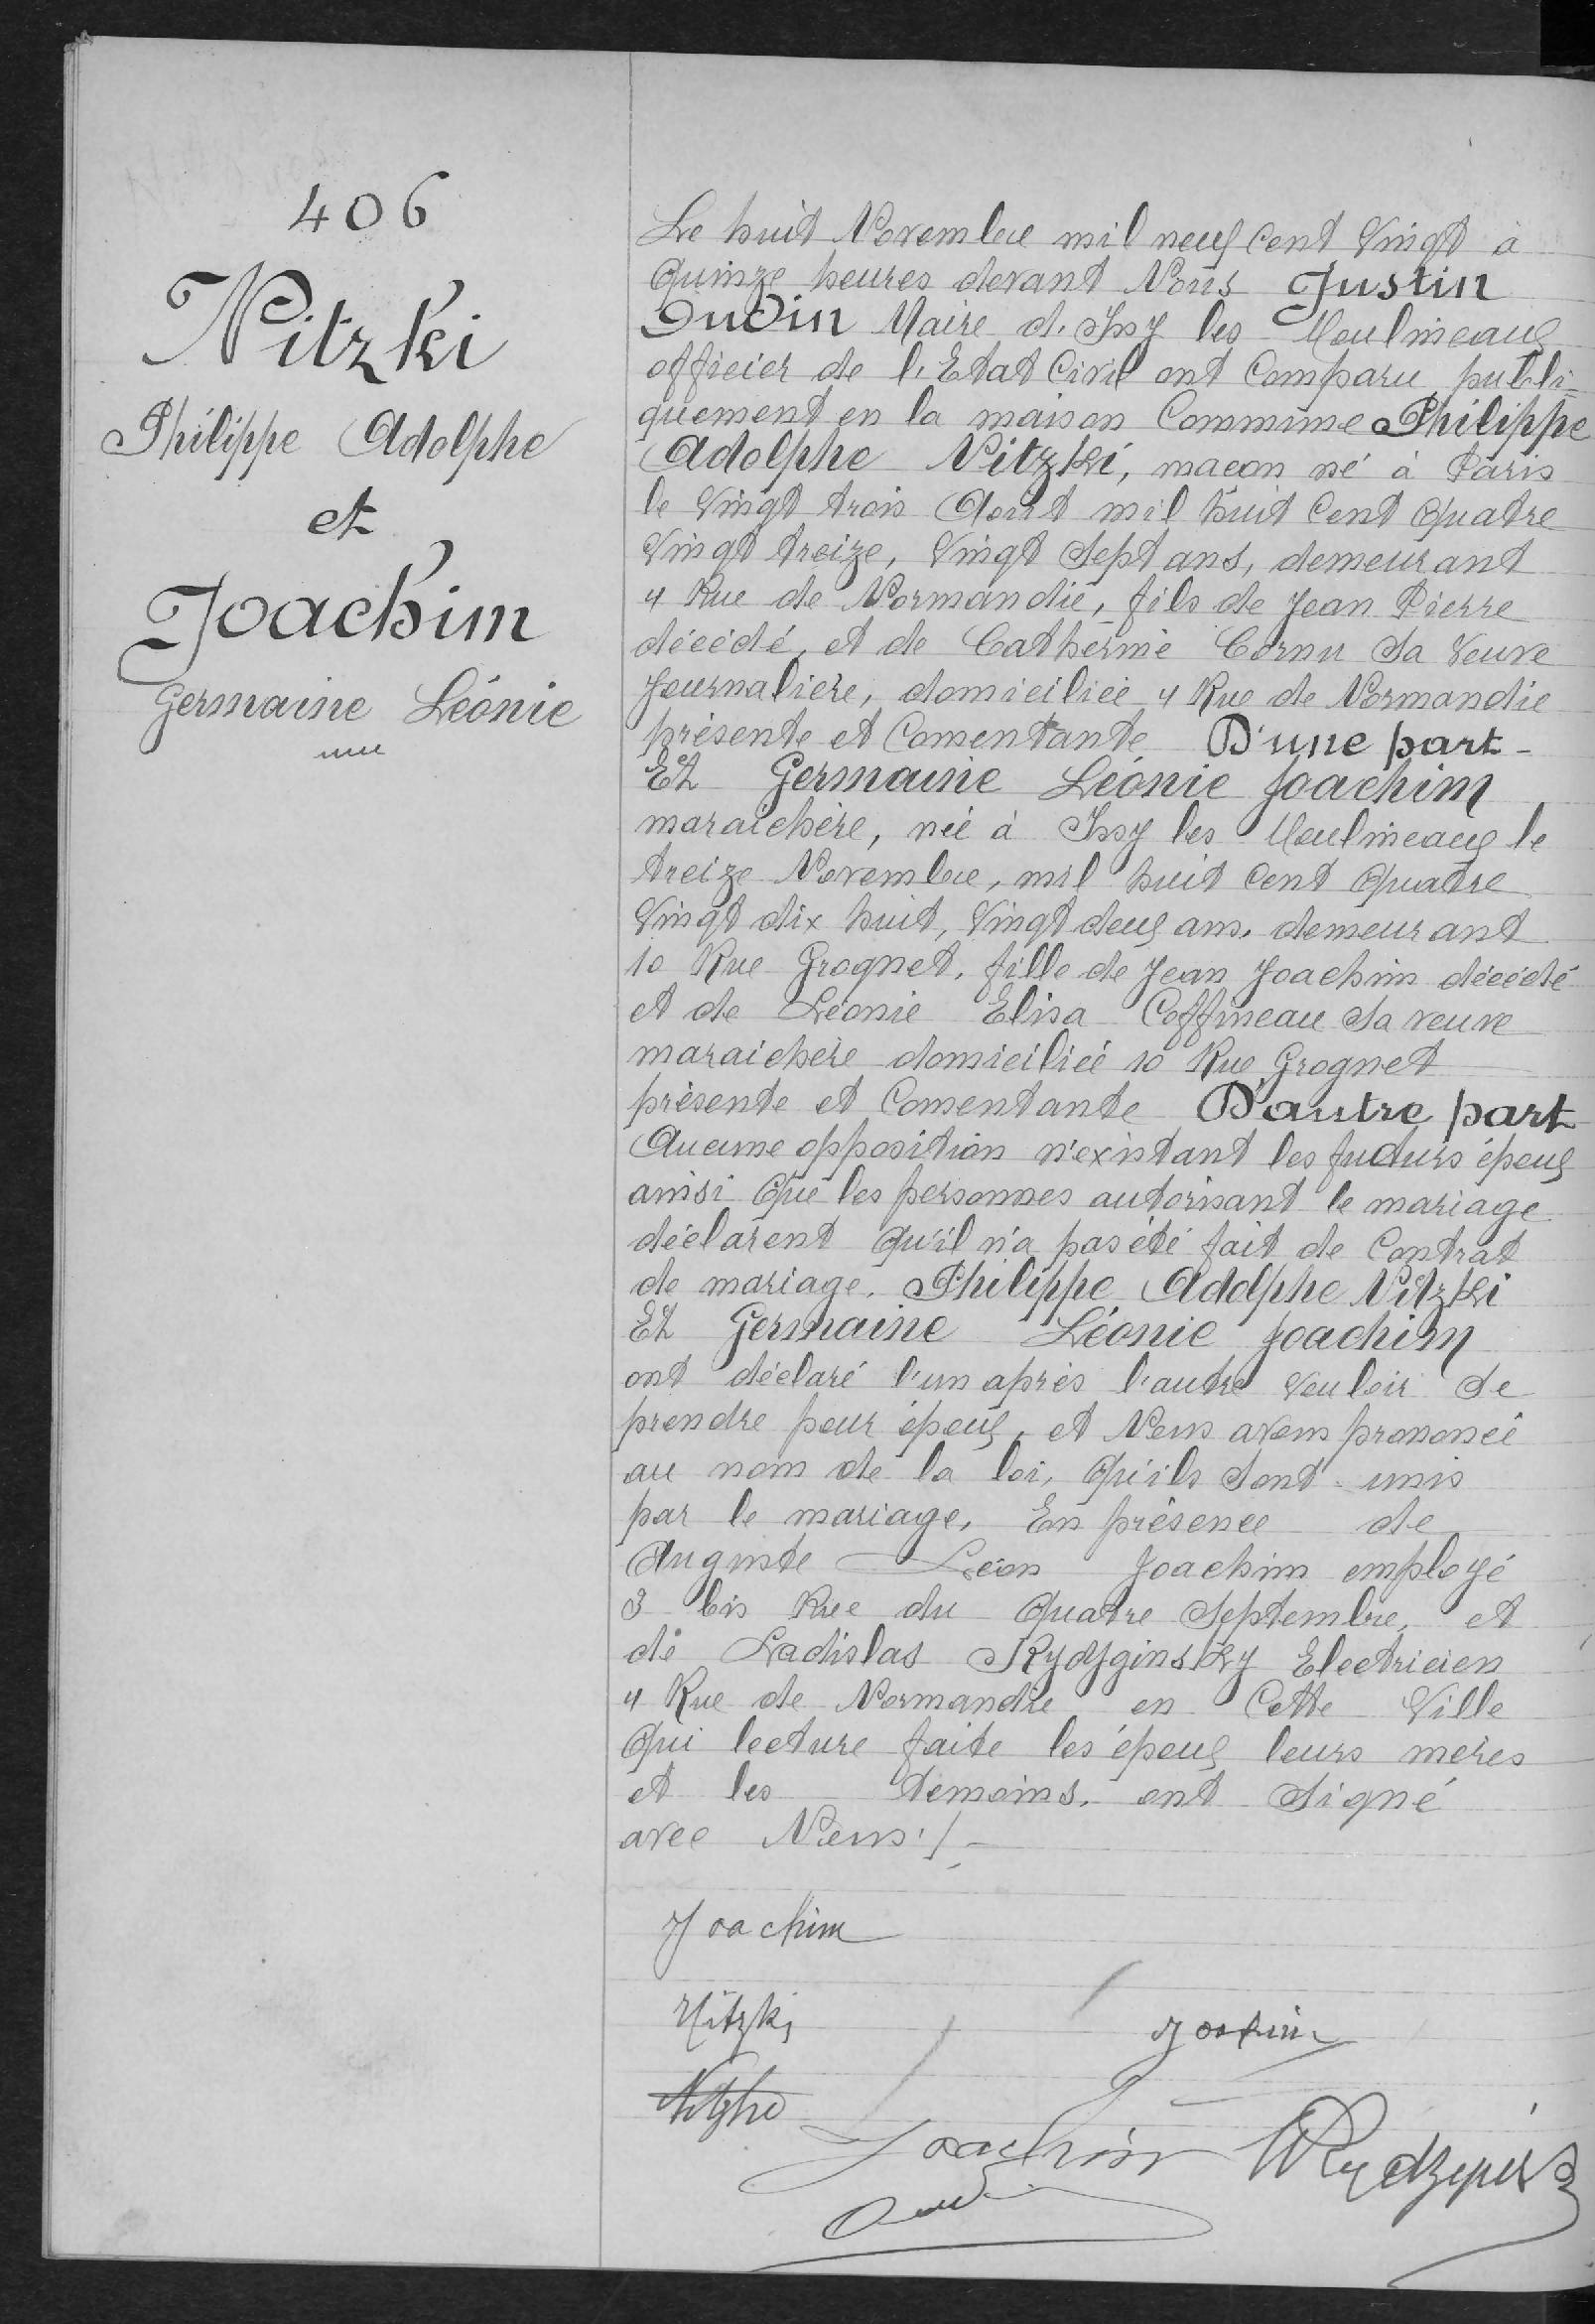406
Nitzki
Philippe Adolphe
et
Joachim
Germaine Léonie
Le huit Novembre mil neuf cent Vingt à
quinze heures devant Nous Justin
Oudin Maire d'Issy les Moulineaux
officier de l'Etat Civil ont comparu publi-
quement en la maison Commune Philippe
Adolphe Nitzki, maçon né à Paris
le vingt trois Avril mil huit cent quatre
vingt treize, vingt sept ans, demeurant
4 Rue de Normandie, fils de Jean Pierre
décédé et de Catherine Cornu sa Veuve
Journaliere, domiciliée 4 Rue de Normandie
présente et Consentante D'une part
Et Germaine Léonie Joachim
maraichère, née à Issy les Moulineaux le
treize Novembre, mil huit cent quatre
vingt dix huit, vingt deux ans, demeurant
10 rue Grognet, fille de Jean Joachim décédé
et de Léonie Elisa Coffineau sa veuve
maraichère domicilié 10 rue Grognet
présente et Consentante D'autre part
Aucune opposition n'existant les futurs époux
ainsi que les personnes autorisant le mariage
déclarent qu'il n'a pas été fait de contrat
de mariage. Philippe Adolphe Nitzki
Et Germaine Léonie Joachim
ont déclaré l'un après l'autre vouloir se
prendre pour époux et Nous avons prononcé
au nom de la loi, qu'ils sont unis
par le mariage, En présence de
Auguste Léon Joachim employé
3 bis Rue du Quatre Septembre et
de Stanislas Ryoyginky Electricien
4 Rue de Normandie en Cette Ville
qui lecture faite les époux leurs mères
et les témoins ont signé
avec Nous ./.
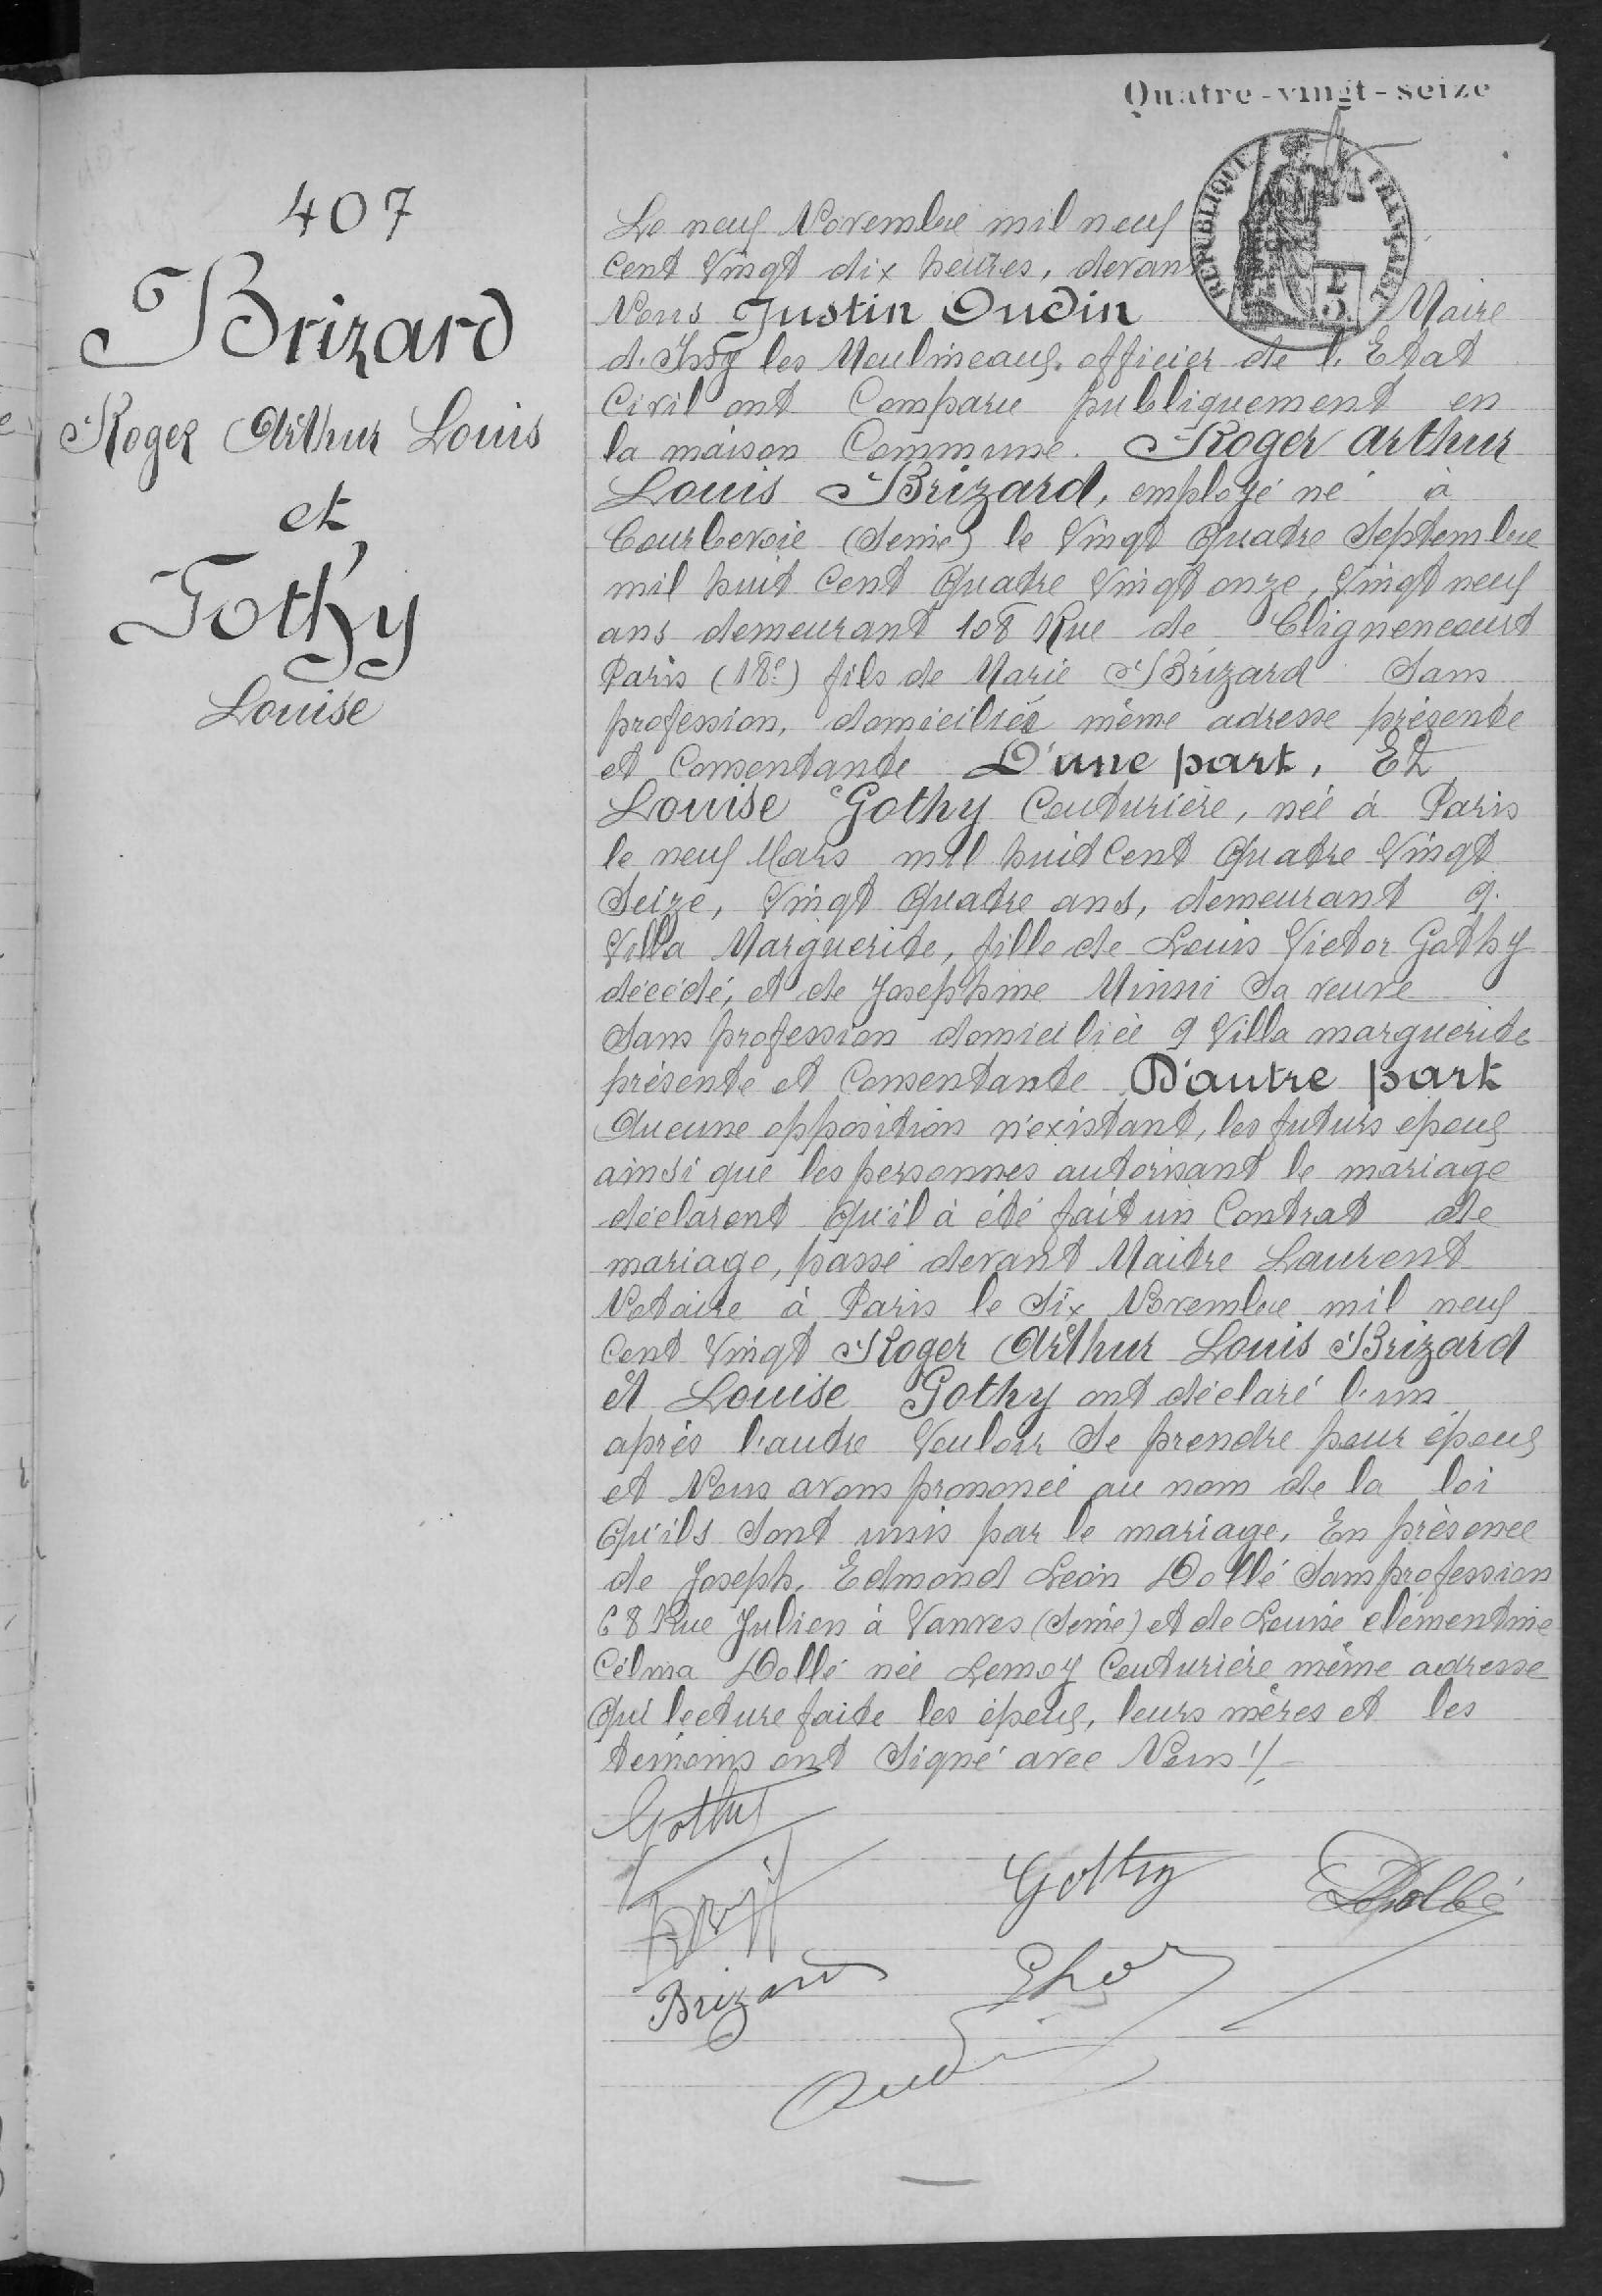407
Brizard
Roger Arthur Louis
et
Gothy
Louise
Le neuf Novembre mil neuf
cent vingt dix heures, devant
Nous Justin Oudin
d'Issy les Moulineaux, officier de l'Etat
Civil ont comparu publiquement en
la maison Commune Roger Arthur
Louis Brizard, employé né à
Courbevoie (Seine) le Vingt quatre septembre
mil huit cent quatre vingt onze vingt neuf
ans demeurant 108 RUe de Clignancourt
Paris (18°) fils de Marie Brézard Sans
profession, domiciliée même aresse présente
et Consentante D'une part. Et
Louise Gothy couturière, née à Paris
le neuf Mars mil huit cent quatre vingt
seize, vingt quatre ans, demeurant 9
Villa Marguerite, fille de Louis Victor Gabby
décédé, et de Joséphine Minni sa veuve.
Sans profession domiciliée 9 Villa marguerite
présente et consentante. D'autre part.
Aucune opposition n'existant, les futurs époux
ainsi que les personne autorisant le mariage
déclarent qu'il a été fait un Contrat de
mariage, passé devant Maitre Laurent
Notaire à Paris le dix Novembre mil neuf
Cent Vingt Roger Arthur Louis Brézard
et Louise Gothy ont déclaré l'un
après l'autre Vouloir se prendre pour époux
et Nous avons prononcé au nom de la loi
qu'ils sont unis par le mariage, En présence
de Joseph, Edmond Léon Dollé sans profession
68 Rue Julien à Vanves (Seine) et de Louise clémentine
Célma Dollé née Lemoy Couturière même adresse
qui lecture faite les époux, leurs mères et les
témoins ont signé avec Nous./.